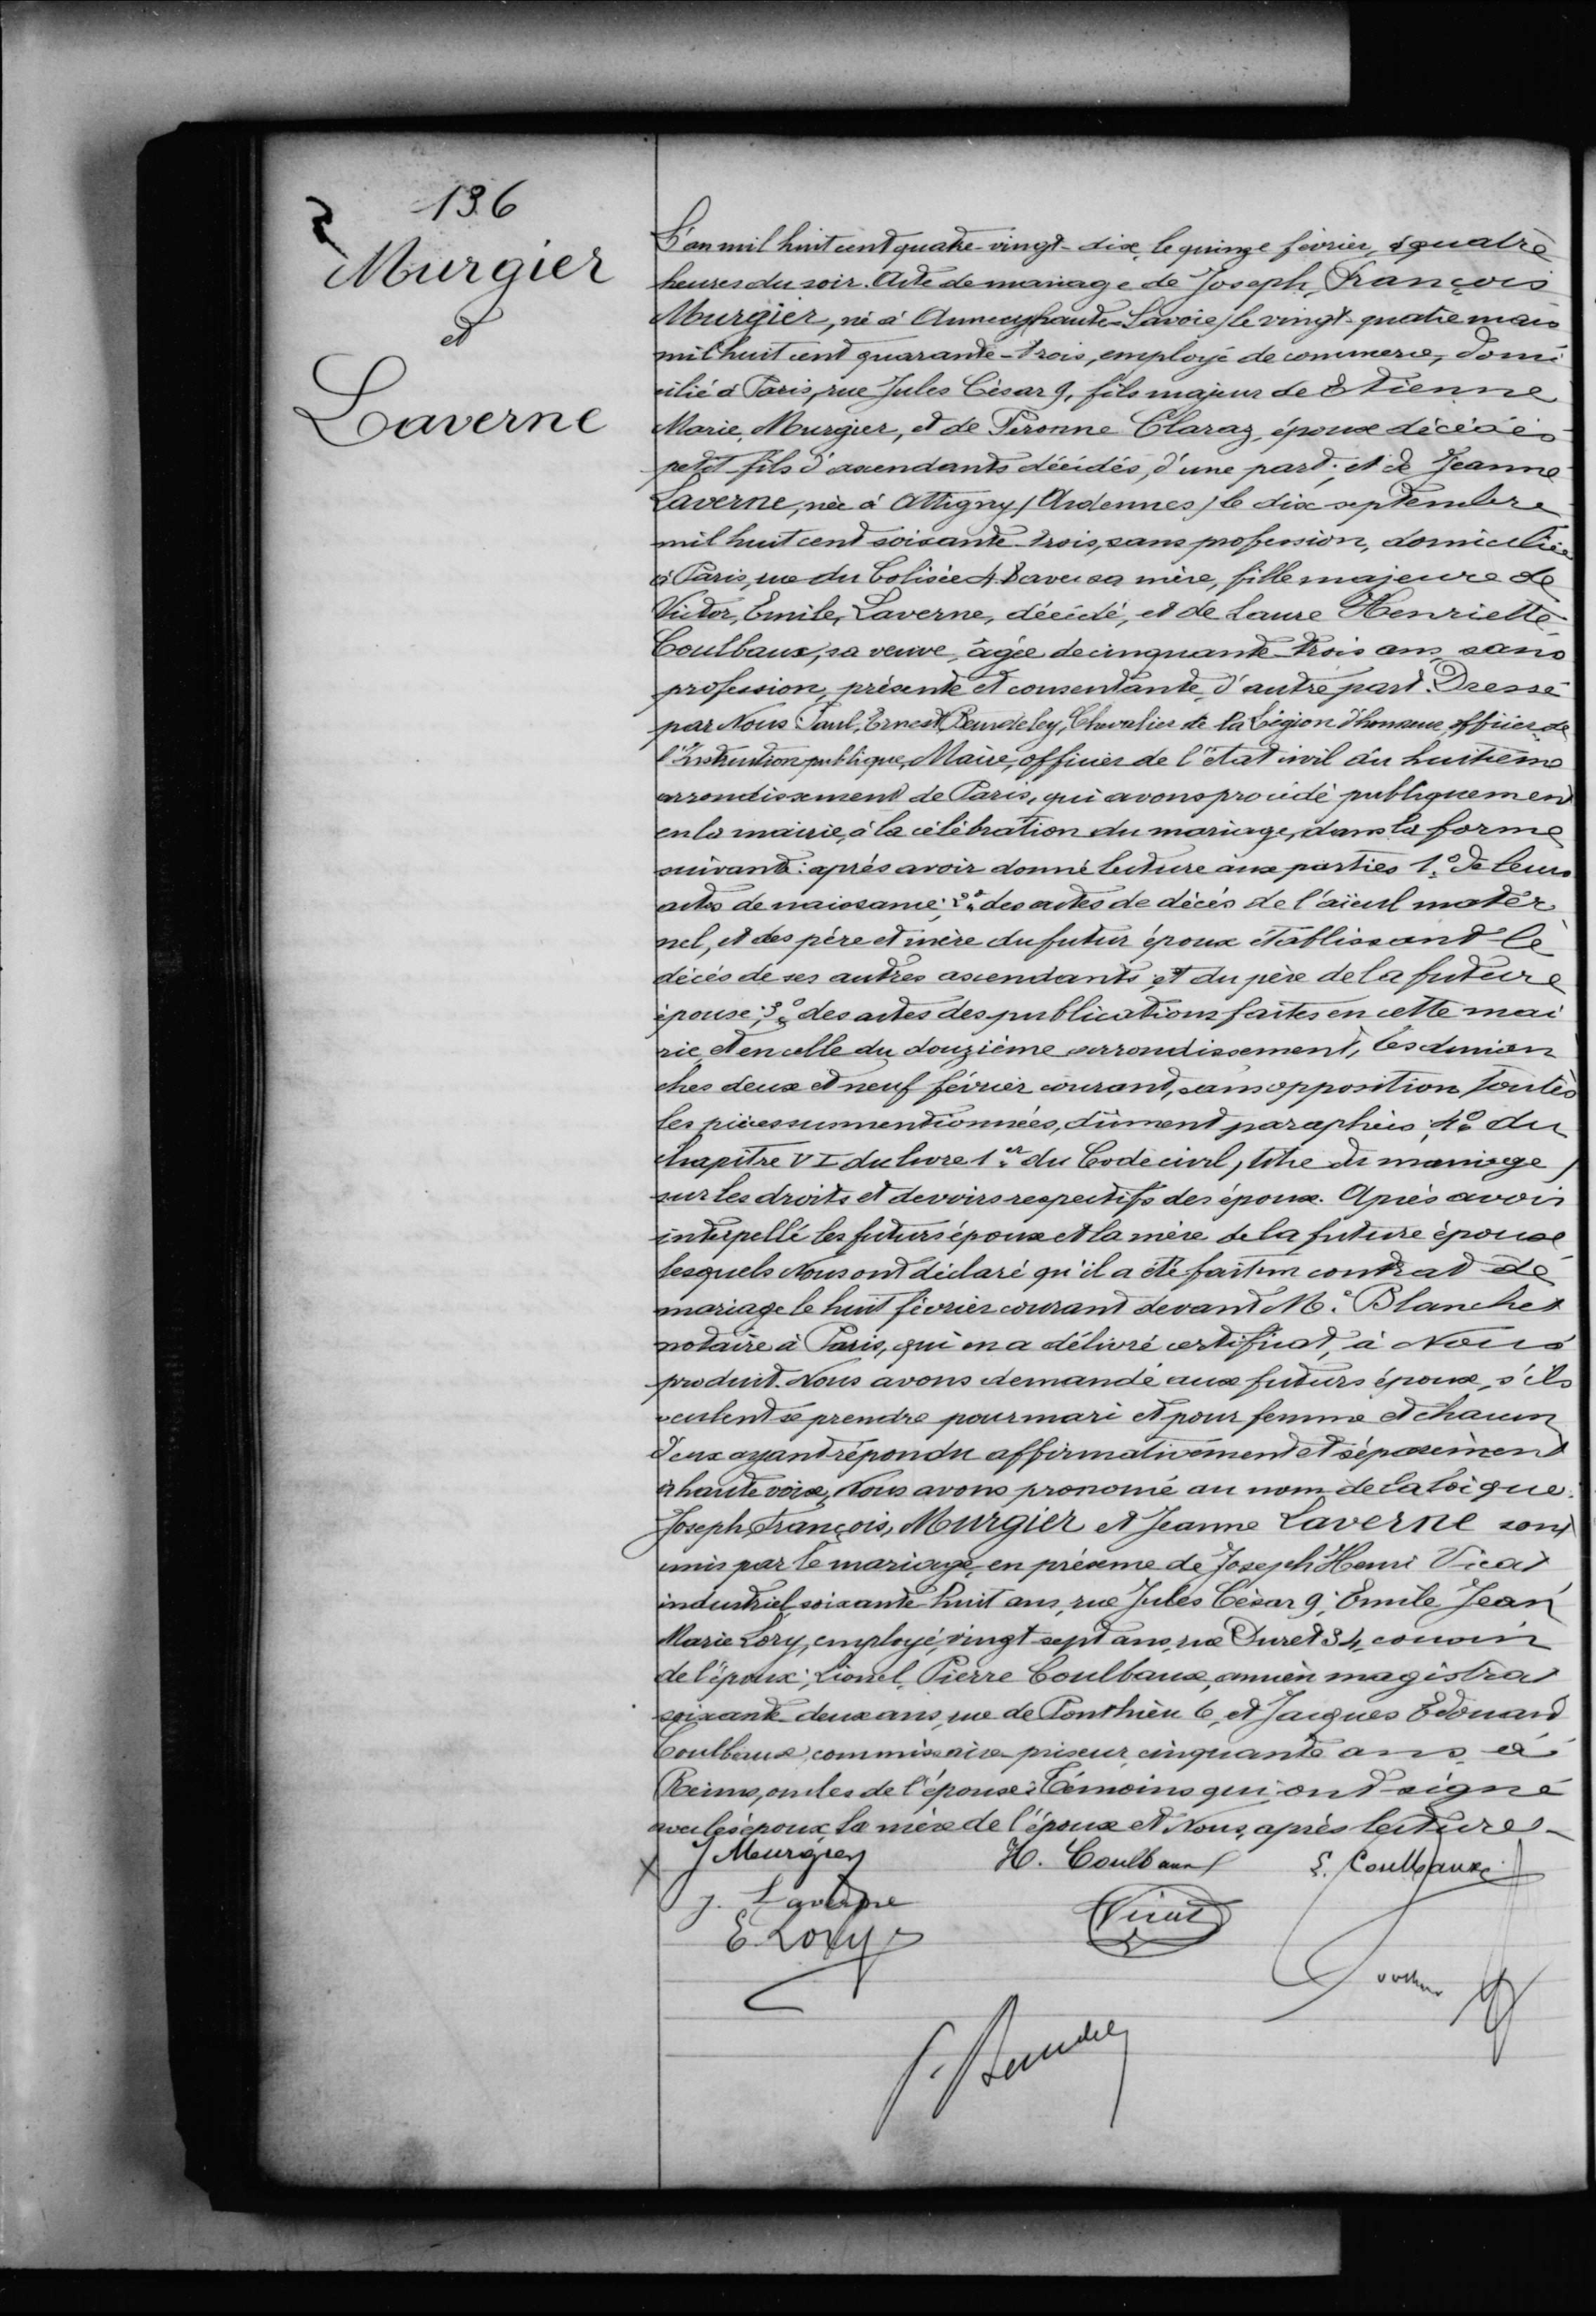136
Murgier
et
Laverne
L'an mil huit cent quatre-vingt-dix, le quinze février à quatre
heures du soir. Acte de mariage de Joseph, François
Aburgier, né à Annecy (Haute-Savoie) le vingt-quatre mai
mil huit cent quarante-trois, employé de commerce, domi
cilié à Paris, rue Jules César 9, fils majeurs de Etienne
Marie Musgier, et de Peronne Claray, épouse décédés
petit-fils d'ascendants décédés, d'une part; et de Jeanne
Laverne, née à Alligny (Ardennes) le dix septembre
mil huit cent soixante-trois, sans profession, domiciliée
à Paris, rue des Colisée 4, avec sa mère, fille majeure de
Victore, Emile, Laverne, décédé, et de Laure Henriette
Coulbaux, sa veuve, âgée de cinquante-trois ans sans
profession, présente et consentante d'autre part. Dressé
par Nous Paul, Ernest, Beurdeley, Chevalier de la Légion d'honneur officier de
l'Instruction publique, Maire, officier de l'état civil du huitième
arrondissement de Paris, qui avons procédé publiquement
en la mairie, à la célébration du mariage, dans la forme
suivante: après avoir donné lecture aux parties 1° de leurs
actes de naissance, 20 des actes de décès de l'aïeul mater
nel, et des père et mère du futur époux établissant le
décès de ss autres ascendants, et du père de la future
épouse; 3° des actes des publications faites en cette mai
rie et en celle du douzième arrondissement, les diman
ches deux et neuf février courant, sans opposition toutes
les pièces susmentionnées, dûment paraphées, 4° du
chapitre VI du livre 1er des Code civil, titre du mariage,
sur les droits et devoirs respectifs des époux. Après avoir
interpellé les futurs époux et la mère de la future épouse
lequels Nous ont déclaré qu'il a été fait un contrat de
mariage le huit février courant devant M° Blanches
notaire à Paris, qui on a délivré certificat, à Nous
produit. Nous avons demandé aux futurs époux, s'ils
veulent se prendre pour mari et pour femme et chacun
d'eux ayant répondu affirmativement et séparément
à haute voix, Nous avons prononcé au nom de la loi que
Joseph François, Murgier et Jeanne Laverne, sont
unis par le mariage, en présence de Joseph Henri Vicat
industriel soixante huit ans, rue Jules César 9; Emile Jean
Marie Lory, employé, vingt sept ans, rue Duret 34, cousin
de l'époux; Lionel Pierre Coulbaux, ancien magistrat
soixante-deux ans, rue de Ponthère 6, et Jacques Edouard
Coulbeux commissaire priseur cinquante ans à
Reims, oncles de l'épouse; Témoins qui ont signé
avec les époux, la mère de l'épouse et Nous après lectures.
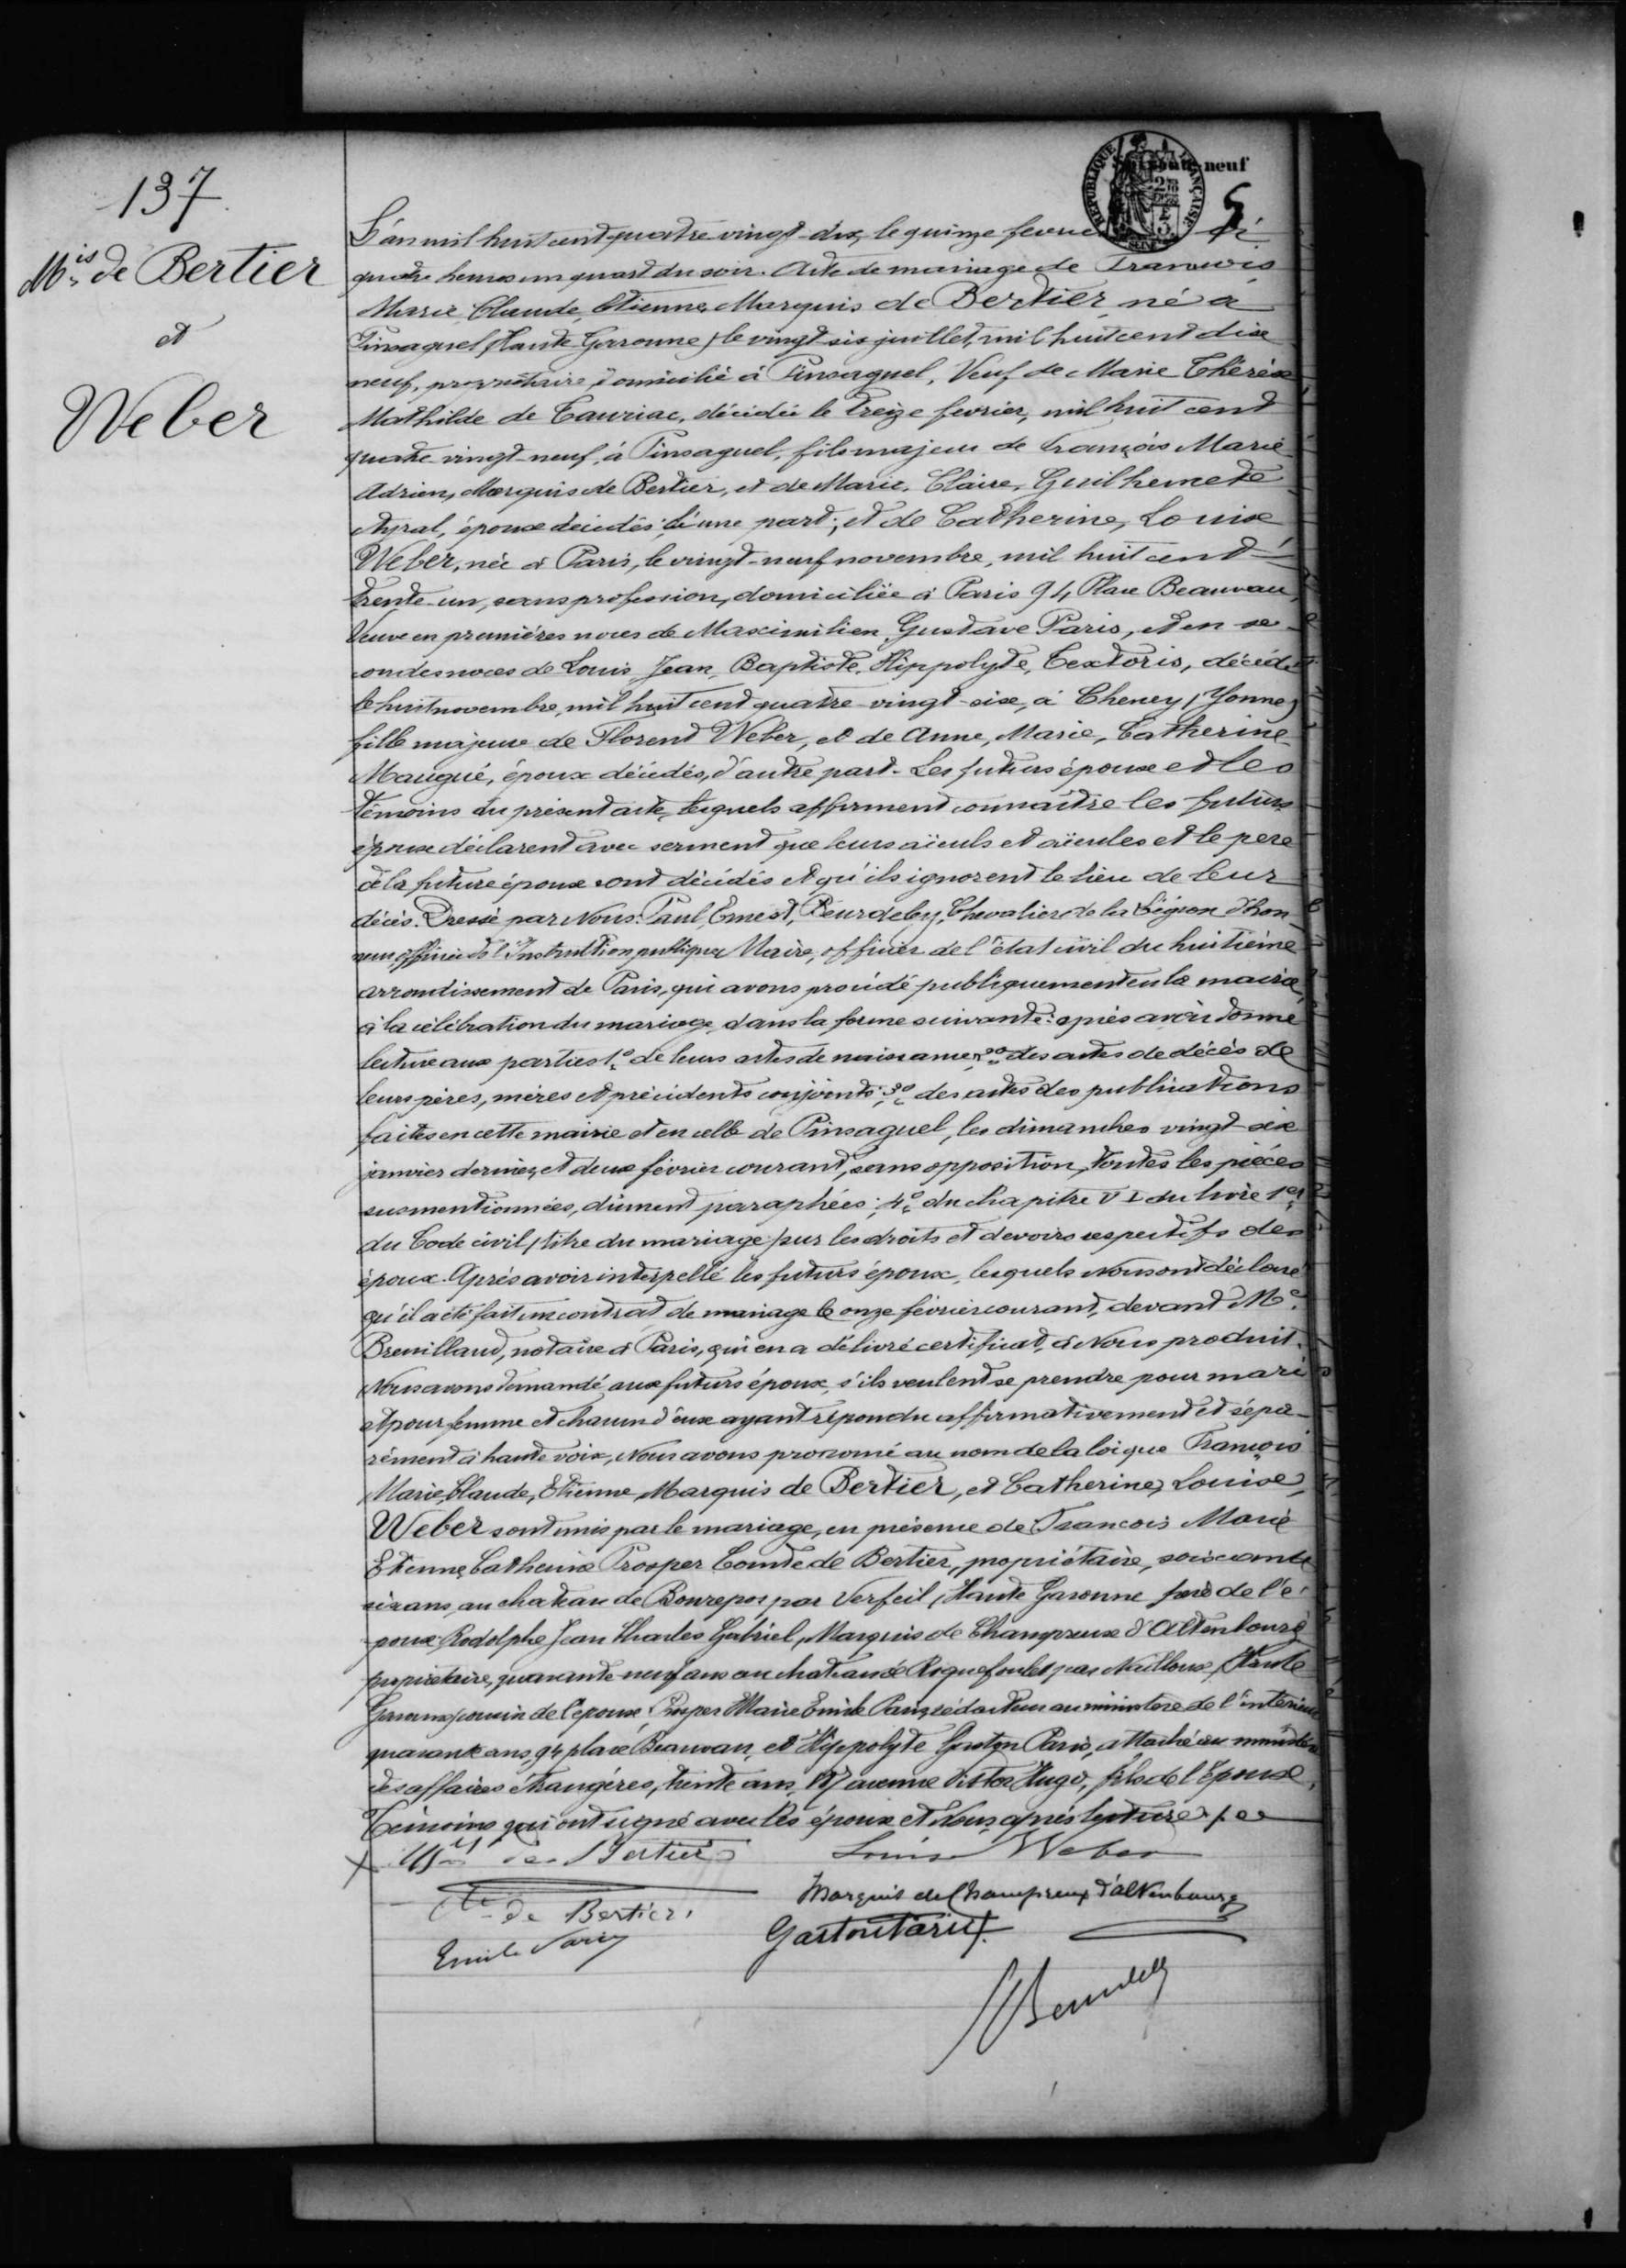137
M de Bertier
et
Welber
L'an mil huit cent quatre vingt-dix, le quinze février
quatre heures un quart sur soir. Acte de mariage de François
Marie Claude, Etienne Marquis de Bertier, né à
Pinoaguel (Haute Garonne) le vingt six juillet mil huit cent dix
neuf, propriétaire, domicilié à Pinoerguel, Veuf de Marie Thérèse
Mathilde de Tauriac, décédée le treize février, mil huit cent
quatre-vingt-neuf, à Pinoarguel, fils majeur et François Marie
Adrien, Marquis de Bestier, et de Marie, Claire, Guilheme de
Ayral, épouse décédés, d'une part; et de Catherine, Louise
Weber, née à Paris, le vingt-neuf novembre, mil huit cent
trente-un, sans profession, domiciliée à Paris 94 Place Beauveau
Veuve en premières noces de Maximilien, Gustave Paris, et en se-
conde noces de Louis Jean Baptiste, Hippolyte, Textoris, décédé
le huit novembre mil huit cent quatre-vingt six, à Chevry (Yonne)
fille majeure de Florent Weber, et de Anne, Marie Catherine.
Maugué, époux décédés, d'autre part. Les futurs époux et les
témoins du présent actes, lesquels affirment connaitre les futurs
époux déclarent avec serment que leurs aïeuls et aïeules et le pere
de la future épous sont décédés et qu'ils ignorent le lieu de leur
décès. Dressé par Nous: Paul Ernest Beurdeby, Chevalier de la Légion d'hon
neuf officier de l'Instruction publique Maire, officier de l'état civil du huitième
arrondissement de Paris, qui avons procédé publiquement en la mairie
à la célébration du mariage, dans la forme suivante: après avoir donné
lecture aux parties 1° de leurs actes de naissance 2° des actes de décés de
leurs pères, mères et précédents conjoints 3° des actes de publications
faites en cette mairie et en celle de Pinoaguel, le dimanche vingt-dix
janvier dernier, et deux février courant, sans opposition, toutes les pièces
susmentionnées, dûment paraphées; 4° du chapitre V du livre 1 er
du Code Civil, titre du mariage pour les droit et les devoirs respectifs des
époux. Après avoir interpellé les futurs époux, lesquels nous ont déclaré
qu'il a été fait un contrat de mariage le onze février courant, devant Me
Brenvillaud, notaire à Paris, qui en a délivré certificat, de Nous produit
Nous avons demandé aux futurs époux s'ils veulent se prendre pour mari
et pour femme et chacun d'eux ayant répondu affirmativement et sépa-
rément à haute voix Nous avons prononcé au nom delaloi que François
Marie, Claude, Etienne, Marquis de Bertier, et Catherine, Louis,
Weber, sont unis par le mariage en présence de François Marie
Etienne, Catherine Prosper Conmte de Bertier, propriétaire, soixante
six ans, au chateau de Bonrepos par Verfeil (Haute Garonne frère de l'é
-poux, Rodolphe Jean Charles Gabriel, Marquis de Chagreux d'Allenbourg
propriétaire, quarante neuf ans au chateau de Riquefoules par Nailloux, (Haute
garonne) cousin de l'épouse, Proser Marie Emile Pasis, rédacteur au ministère de l'intérieur
quarante ans, 94 place Beauvau et Hypolyte Gaston Pasis, attaché de ministère
des affaires étrangères, trente ans, 187 avenue Victor Hugo, fils de l'épouse,
Témoins qui ont signé avec les époux et Nous après lecture.